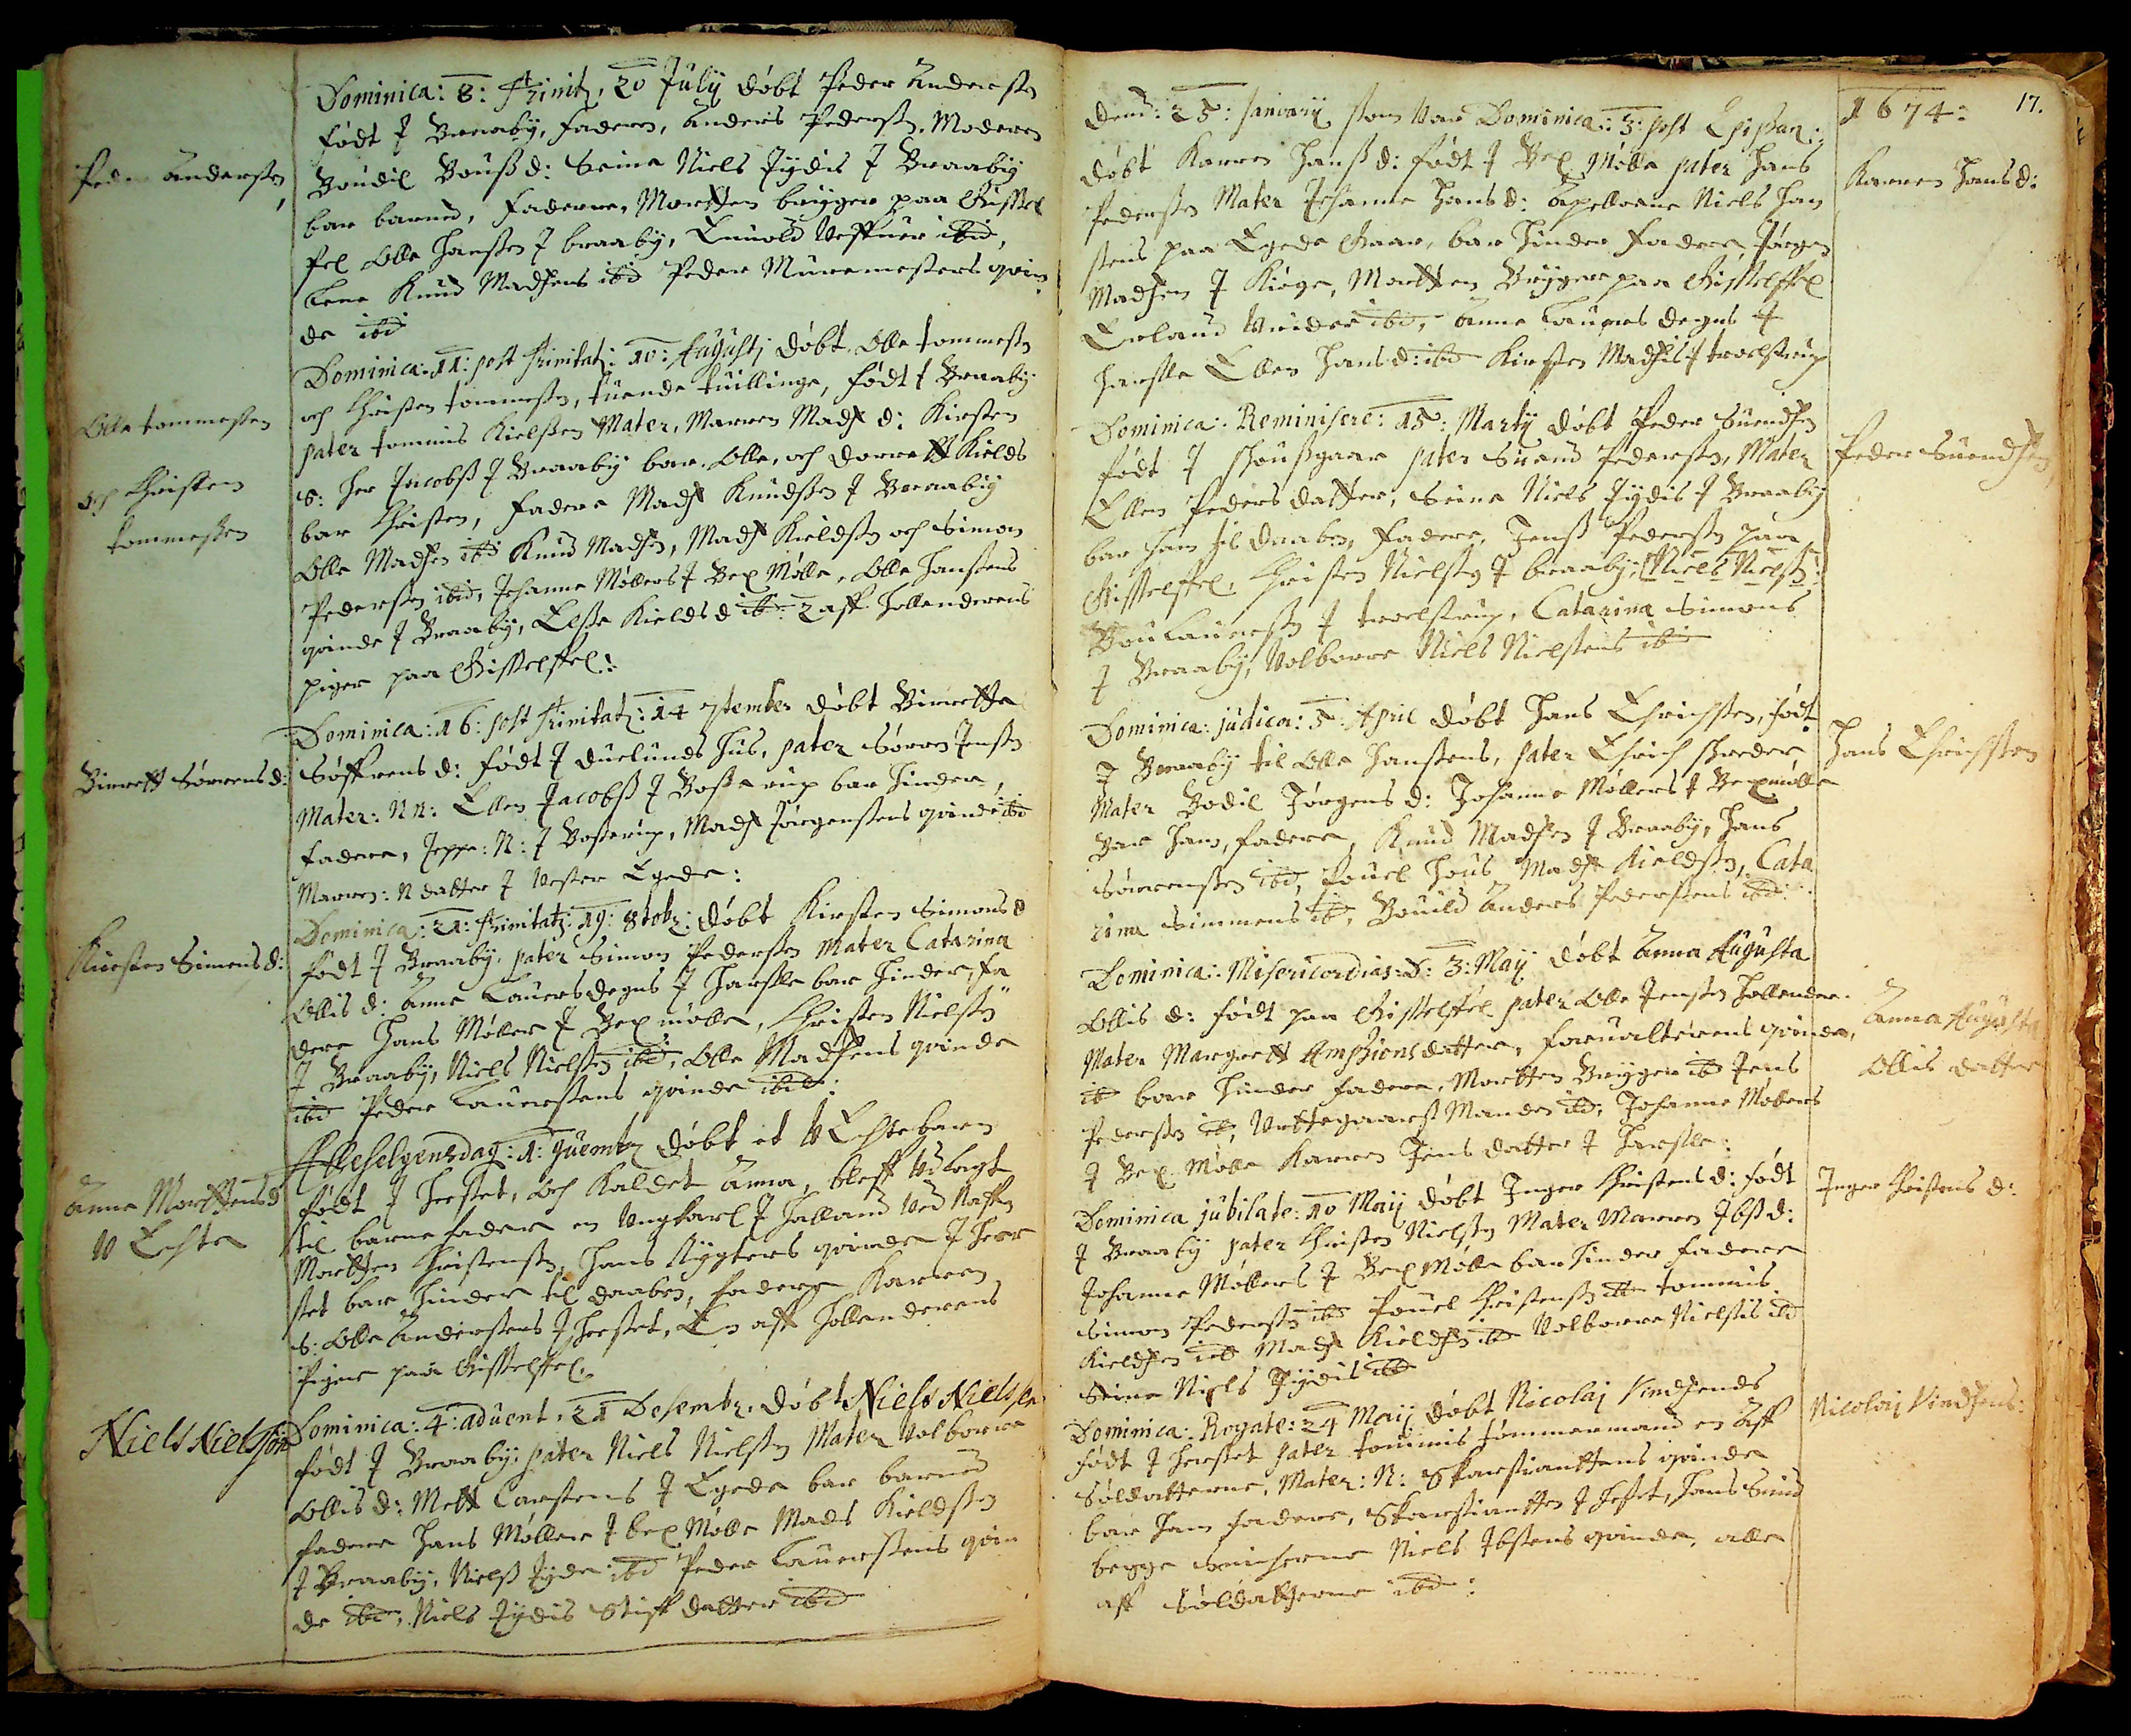Peder Andersen 
Dominica: 8: Trinit, 20 July døbt Peder Andersen
født J Braaby, Faderen Anders Pedersen. Moderen
Boudil Bous d: Seine Niels Jydis J Braaby
bar barned, Faderne, Mortten bryger paa Gissel
fel, Olle Hansen J Braaby, Eneuold Veffuer ibid
Lene Knud Madsens ibid, Peder Muremesters qvin
de ibid.
Olle Tommesen 
och Christen 
Tommesen 
Dominica: 11: post Trinitat: 10: Augustj døbt Olle Tommesen
och Christen Tommesen, tuende tuillinger, født J Braaby
Pater Tommis Kielsen. Mater, Marren Mads d: Kirsten
S: her Jacobs J Braaby bar. Olle, och Dorrett Kields
bar Christen, Fadere Mads Knudsen J Braaby.
Olle Madsen ibd. Knud Madsen, Mads Kieldsen och Simon
Pedersen ibid, Johanne Møllers J Bex Mølle, Olle Hansens
qvinde J Braaby, Else Kields d. ibid. 2 aff Hollenderens
piger paa Gisselffel:
Birrett Sørrens d: 
Dominica: 16: post Trinitat: 14 7tember døbt Birrette
Søffrens d: født J Duelunds hus, Pater Sørren Jensen
Mater: N N: Ellen Jacobsen J Boserup bar hinder,
Fadere Jeppe: N: J Boserup, Mads Jørgensens qvinde ibd.
Marren: N: datter J Vester Egede:
Kirsten Simons d: 
Dominica: 21: Trinitatz: 19: 8tobr: døbt Kirsten Simons d.
født J Braaby, Pater Simon Pedersen, Mater Catarina
Ollis d: Anne Lauers degns J Harsle bar hinder, Fa¬
dere Hans Møller J Bex mølle, Christen Nielsen
J Braaby, Niels Nielsen ibd. Olle Madsens qvinde
ibd. Peder Lauersens qvinde ibid:
Anne Morttens d. 
Uechte 
Allehelgenesdag: 1: 9uembz## døbt et U Echte barn
født J Heeset, och kaldet Anna, bleff Udlagt
til barneFader en Ungkarl i Halland ved Naffen
Mortten Christensen, Hans Rygters qvinde J Her
set bar hinder til daaben, Fadere Karren
S: Olle Andersens J Herset, En aff Hollenderens
Piger paa Gisselfel.
Niels Nielsen 
Dominica: 4: aduent. 21 Desembr døbt Niels Nielsen
født J Braaby: Pater Niels Nielsen, Mater Valborre
Ollisd: Mette Carstens J Egede bar barned
Fadere Hans Møller J Bex Mølle, Mads Kieldsen
J Braaby, Niels Jyde ibd. Peder Lauersens qvin
de ibd. Niels Jydis, Stiff datter ibd.
Dend: 25: Janoary som var Dominica: 3. post Epiphan:
døbt Karren Hans d: født J Bex Mølle Pater Hans
Pedersen, Mater Johanne Hansd: Apelloene Niels Hans
sens paa Egede Gaar, bar hinder. Fadere Jørgen
Madsen J Kiøge, Mortten Bryger paa Gisselffel
Erland Urider## ibd. Anne Lauers degns af
Harsle, Ellen Hans d: ibd Kirsten Madsis J Troelstrup.
17.
1674
Karren Hans d:
Dominica: Reminisere: 15: Marty døbt Peder Suendsen
født J Skousgaar. Pater Suend Pedersen, Mater
Ellen Peders datter, Seine Niels Jydis J Braaby
bar ham til daaben. Fadere Jens Pedersen paa
Gisselfel. Christen Nielsen J Braaby: ##
Bou Lauersen J Troelstrup, Catarina Simons
J Braaby, Valborre, Niels Nielsen ibd.
Peder Suendsen
Dominica: judica: 5. April døbt Hans Ehrichsen, født
J Braaby til Olle Hansens, Pater Ehrich Skreder,
Mater Bodil Jørgens d: Johanne Møllers J Bexmølle
Bar Ham, Fadere Knud Madsen i Braaby, Hans
Sørrensen ibd. Pouel Hous, Mads Kieldsen, Cata
rina Simmons ibd. Bouild Anders Pedersens ibd:
Hans Ehrichsen
Domiinica: Misericordias d: 3: May døbt Anna Augusta
Ollis d: født paa Gisselfel. Pater Olle Jensen Hollender.
Mater Margrett Ampzionsdatter, forualterens qvinde
ib bar hinder Fadere Mortten Bryger ibd. Jens
Pedersen ibd. Urttegaars Manden ibd. Johanne Møllers
J Bex Mølle, Karren Jens datter J Harsle:
Anna Augusta 
Ollis datter 
Dominica jubilate: 10 May døbt Jnger Christens d: født
J Braaby. Pater Christen Nielsen Mater Marren Jbsd:
Johanne Møllers J Bex Mølle bar hinder. Fadere
Simon Pedersen ibd. Pouel Christensen ibd. Tommis
Kieldzen ibd. Mads Kieldzen ibd. Valborre Nielsis ibd.
Seine Niels Jydis ibd.
Jnger Christens d: 
Dominica: Rogate: 24 May døbt Nicolai Vindsends##
født J Herset. Pater Tommis Tømmermand en Aff
Søldatterne, Mater: N: Skarsianttens qvinde
bar ham, Fadere:Skarsiantten J Heset, Hans Smid
begge snicheren Niels Jbsens qvinde alle
aff Søldatterne ibid:
Nicolai Vindseids:
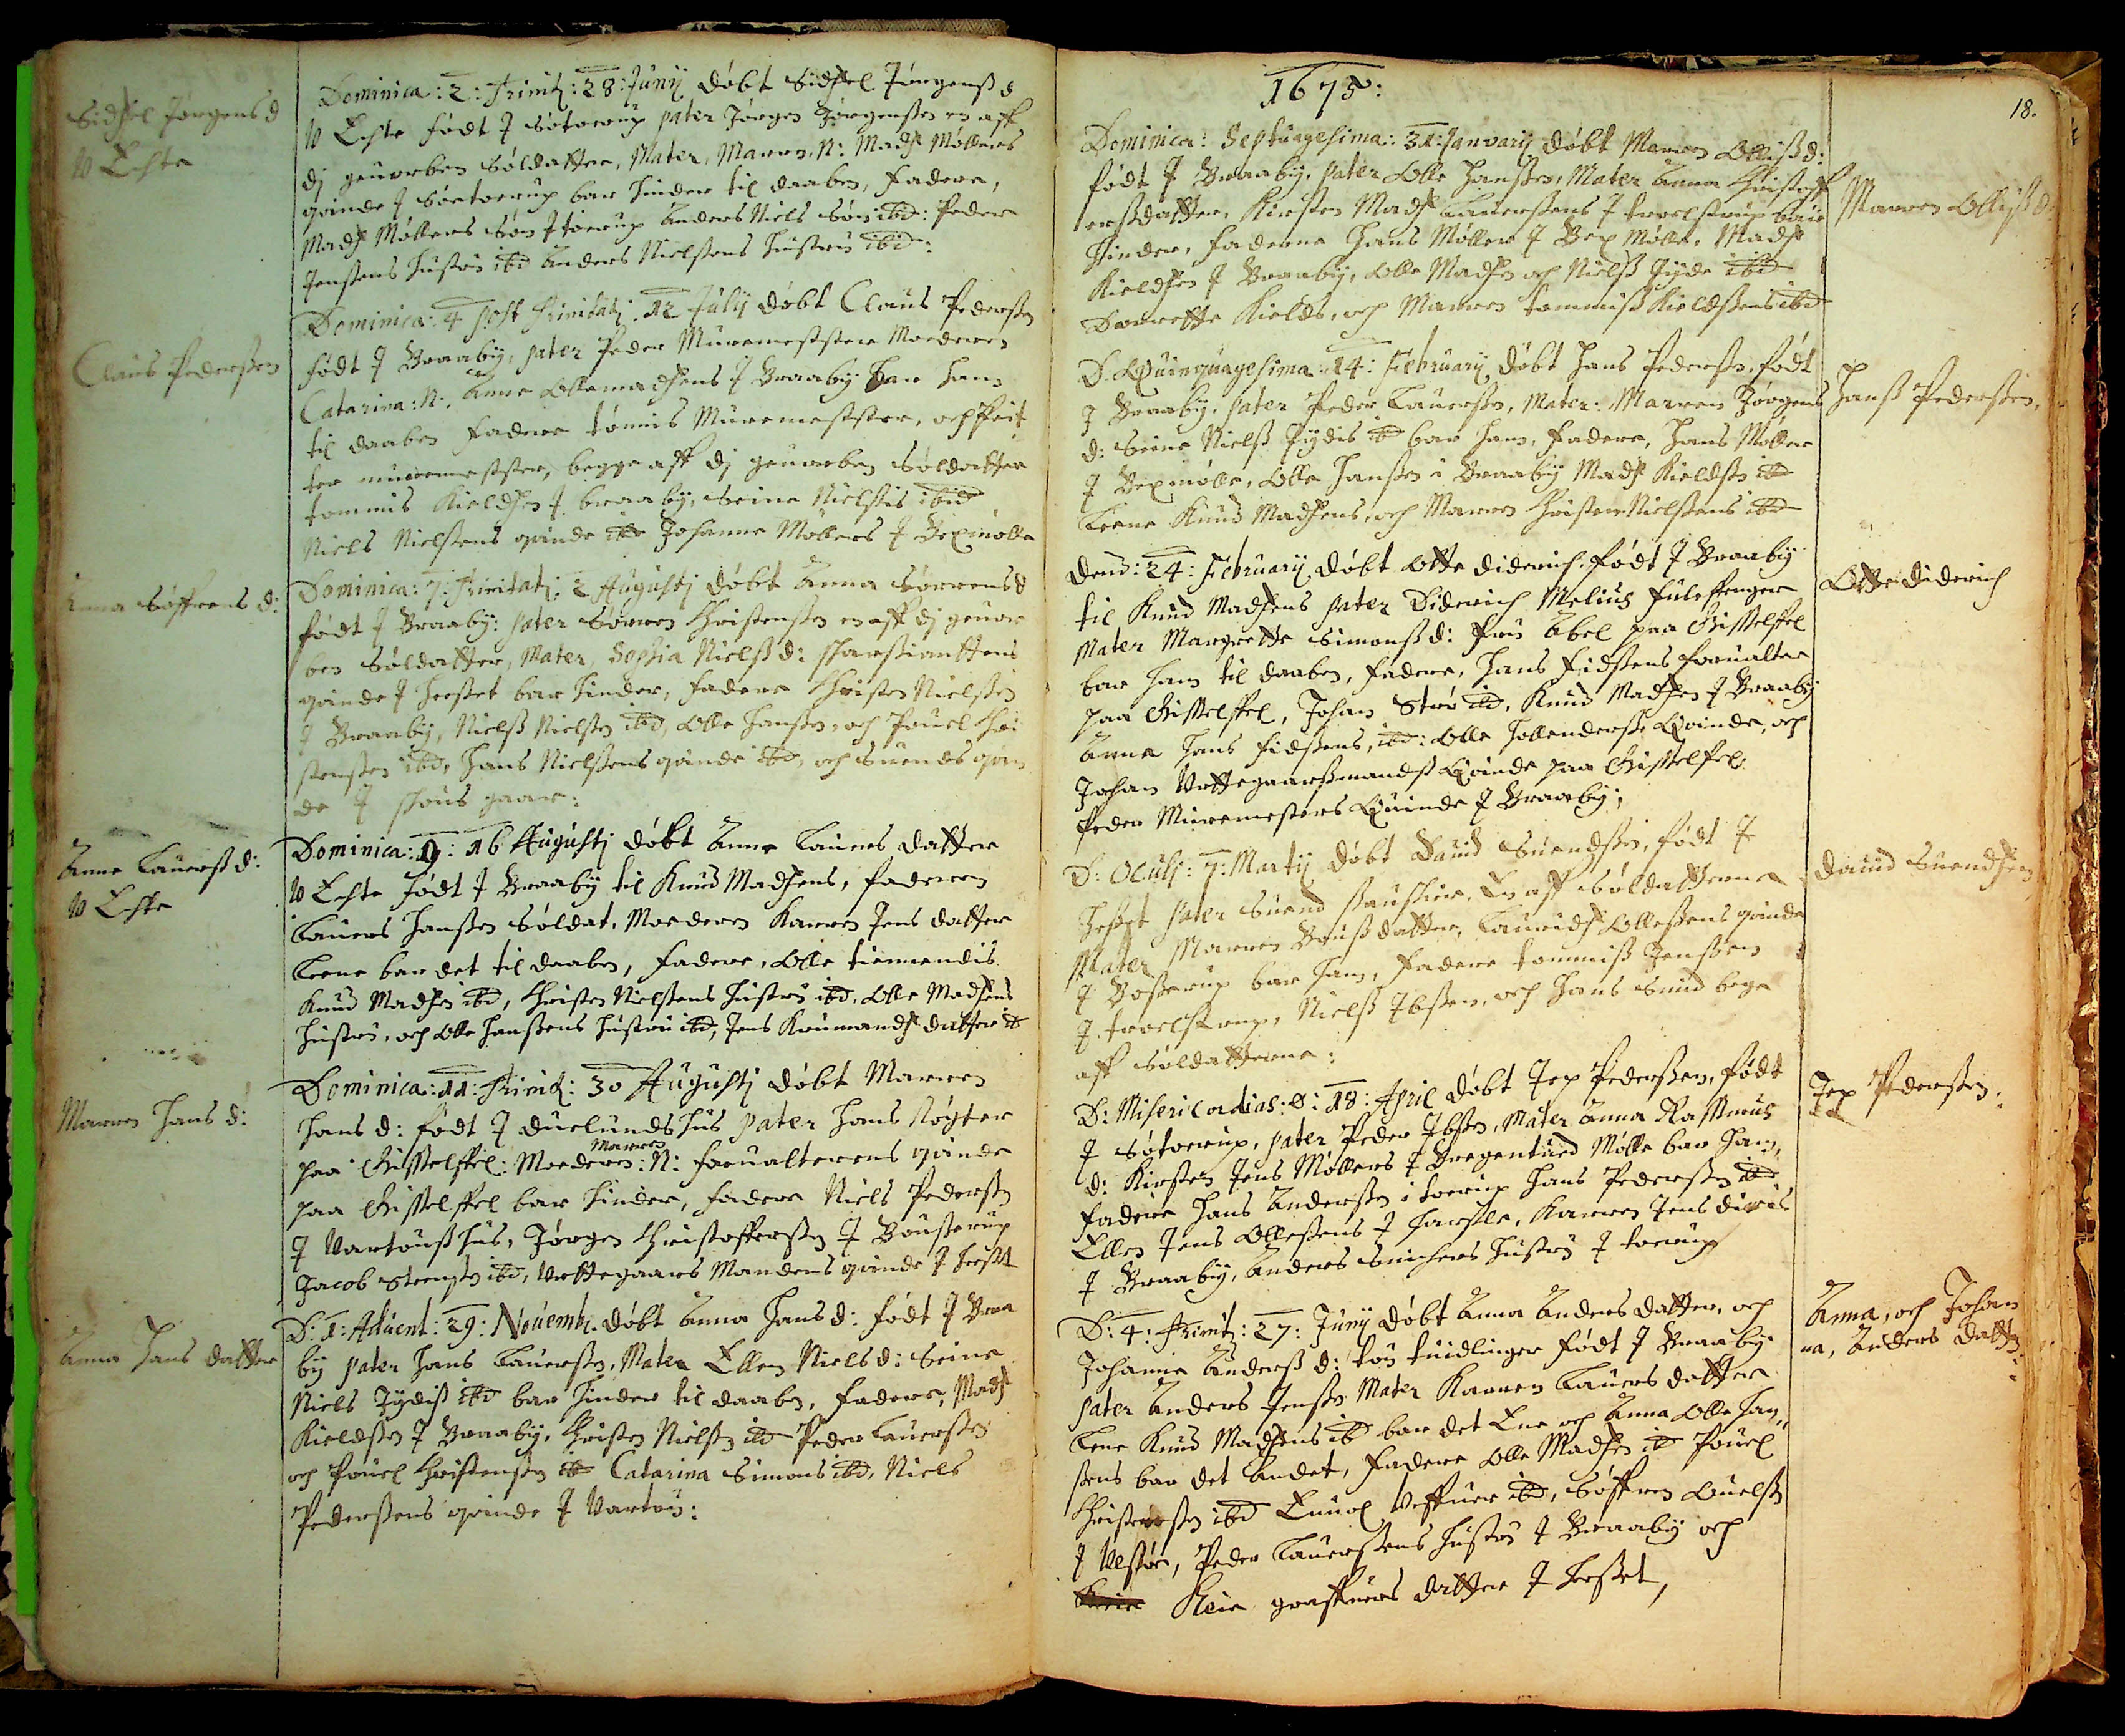Sidsel Jørgens d. 
U Echte 
Dominica: 2: Trinit: 28: Juny døbt Sidsel Jørgens d
U Echte født J Søtoerup Pater Jørgen Jørgensen en aff
dj geuorben Søldatter, Mater Marren N: Mads Møllers
qvinde J Søtoerup bar hinder til daaben, Fadere,
Mads Møllers Søn J Toerup, Anders Niels Søn ibd.: Peder
Jensens hustru ibd. Anders Nielsens hustru ibd:
Claus Pedersen 
Domiinica: 4 post Trinitatz##. 12 July døbt Claus Pedersen
født J Braaby, Pater Peder Muremestster. Moderen
Catarina: N: Anne Olle Madsens J Braaby bar ham
til daaben. Fadere Tønnis Muremestster, och Peit
ter Muremestster begge aff dj geureben Søldatter
Tommis Kielssen J Braaby. Seine Nielsen ibid.
Niels Nielsen qvinde ibd. Johanne Møllers J Bexmølle.
Anna Sørrensd:
Dominica: 7:Trinitatz 2 Augustj døbt Anna Sørrensd:
født J Braaby: Pater Sørren Christensen en aff dj geuor
ben Søldatter, Mater Sophia Niels d: Skarsianttens
qvinde J Heeset bar hinder, Fadere Christen Nielsen
J Braaby, Niels Nielsen ibd. Olle Hansen, och Pouel Chri
stensen ibd. Hans Nielsens qvinde ibd. och Suends qvin
de J Skousgaar:
Anne Lauers d: 
U Echte 
Dominica: 9: 16 Augustj døbt Anne Lauers datter
U Echte født J Braaby til Knud Madsens, Faderen
Lauers Hansen Søldat, Moderen Karren Jens datter
Leene bar det til daaben, Fadere Olle tiennendis
Knud Madsen ibd. Christen Nielsens Hustru ibd. Olle Madsens
Hustru och Olle Jensens Hustru ibd. Jens Krumands datter ibd.
Marren Hans d: 
Dominica: 11: Trinitz: 30 Augustj døbt Marren
Hans d: født J Duelunds hus. Pater Hans Røgter
Marren
paa Gisselffel: Moederen: N: forualterens qvinde
paa Gisselfeld bar hinder, Fadere Niels Pedersen
J Vartous hus, Jørgen Christoffersen J Bouserup
Jacob Steensen ibd. Urttegaars Mandens qvinde J Heeset.
Anna Hans datter 
D: 1: Aduent: 29: Nouembr. døbt Anna Hans d: født J Braa
by. Pater Hans Lauersen, Mater Ellen Nielsd: Seine
Niels Jydis ibd. bar hinder til daaben. Fadere Mads
Kieldsen J Braaby. Christen Nielsen ibd. Peder Lauersen
och Pouel Christensen ibd. Catarina Simons ibd. Niels
Pedersens qvinde i Vartou:
1675:
Dominica: Septuegesima: 31 Janoary døbt Marren Ollisd:
født J Braaby, Pater Olle Hansen. Mater Anna Christof
fers datter. Kirsten Mads Lauersens J Troelstrup bar
hinder, Faderne Hans Møllers J Bex Mølle. Mads
Kieldzen J Braaby, Olle Madsen och Niels Jyde ibid.
Dorrette Kields, och Marren Tommis Kieldsens ibid.
18.
Marren Ollis d: 
D: Quinpuagesima: 14: Februarij døbt Hans Pedersen, født 
J Braaby, Pater Peder Lauersen, Mater Marren Jørgens 
d: Siine Niels Jydis ibd. bar ham. Fadere, Hans Møller 
J Bexmølle, Olle Hansen i Braaby, Madz Kieldsen ibd. 
Leene Knud Madzens, och Marren Christen Nielsens ibd.
Hans Pedersen 
Dend: 24: February døbt Otte Diderich født J Braaby
til Knud Madsens Pater Diderich Melius fuleffenger
Mater Margrette Simonsd: Fru Abel paa Gisselfel
bar ham til daaben, Fadere, Hans Fidsens forualter
paa Gisselfel, Johan Strur ibd. Knud Madsen J Braaby,
Anna Hans Fidsens, ibd. Olle Hollænders Qvinde, och
Johan Urttegaarsmands Qvinde paa Gisselfel.
Peder Muremesters Quinde J Braaby;
Otte Diderich
D: Oculj: 7: Marty døbt Dauid Suendsen født J
Heset. Pater Suend Sauskier En aff Søldatterne 
Mater Marren Bousdatter, Lauridz Ollesens qvinde
J Boserup bar ham. Fadere Tommis Jensen
J Troelstrup, Niels Jbsen, och Hans Smid bege
aff Søldatterne:
Daiud Suendzen
D: Misericordias: D: 18 April døbt Jep Pedersen, født 
J Søtoerup, Pater Peder Jbsen, Mater Anna Rassmus 
d: Kirsten Jens Møllers J Bregentued Mølle bar ham.
Fadere Hans Andersen i Toerup. Hans Pedersen ibd.
Ellen Jens Ollesens J Harsle. Karren Jens Diris
J Braaby, Anders Snichers Hustru J Toerup
Jep Pedersen
D: 4: Trinitz: 27: Juny Anna Anders datter, och
Johanna Anders d: tou tuidlinger født J Braaby
Pater Anders Jensen Mater Karren Lauers datter
Lene Knud Madsens ibd bar det Ene och Anna Olle Jen¬
sens bar det Andet, Fadere Olle Madsen ibd. Pouel
Christensen ibd. Enuol Veffuer ibd, Søffren Ouelsen
J Uelse, Peder Lauersens Hustru J Braaby och
## Kaie graffuers datter J Herset,
Anna och Johan
na, Anders datter